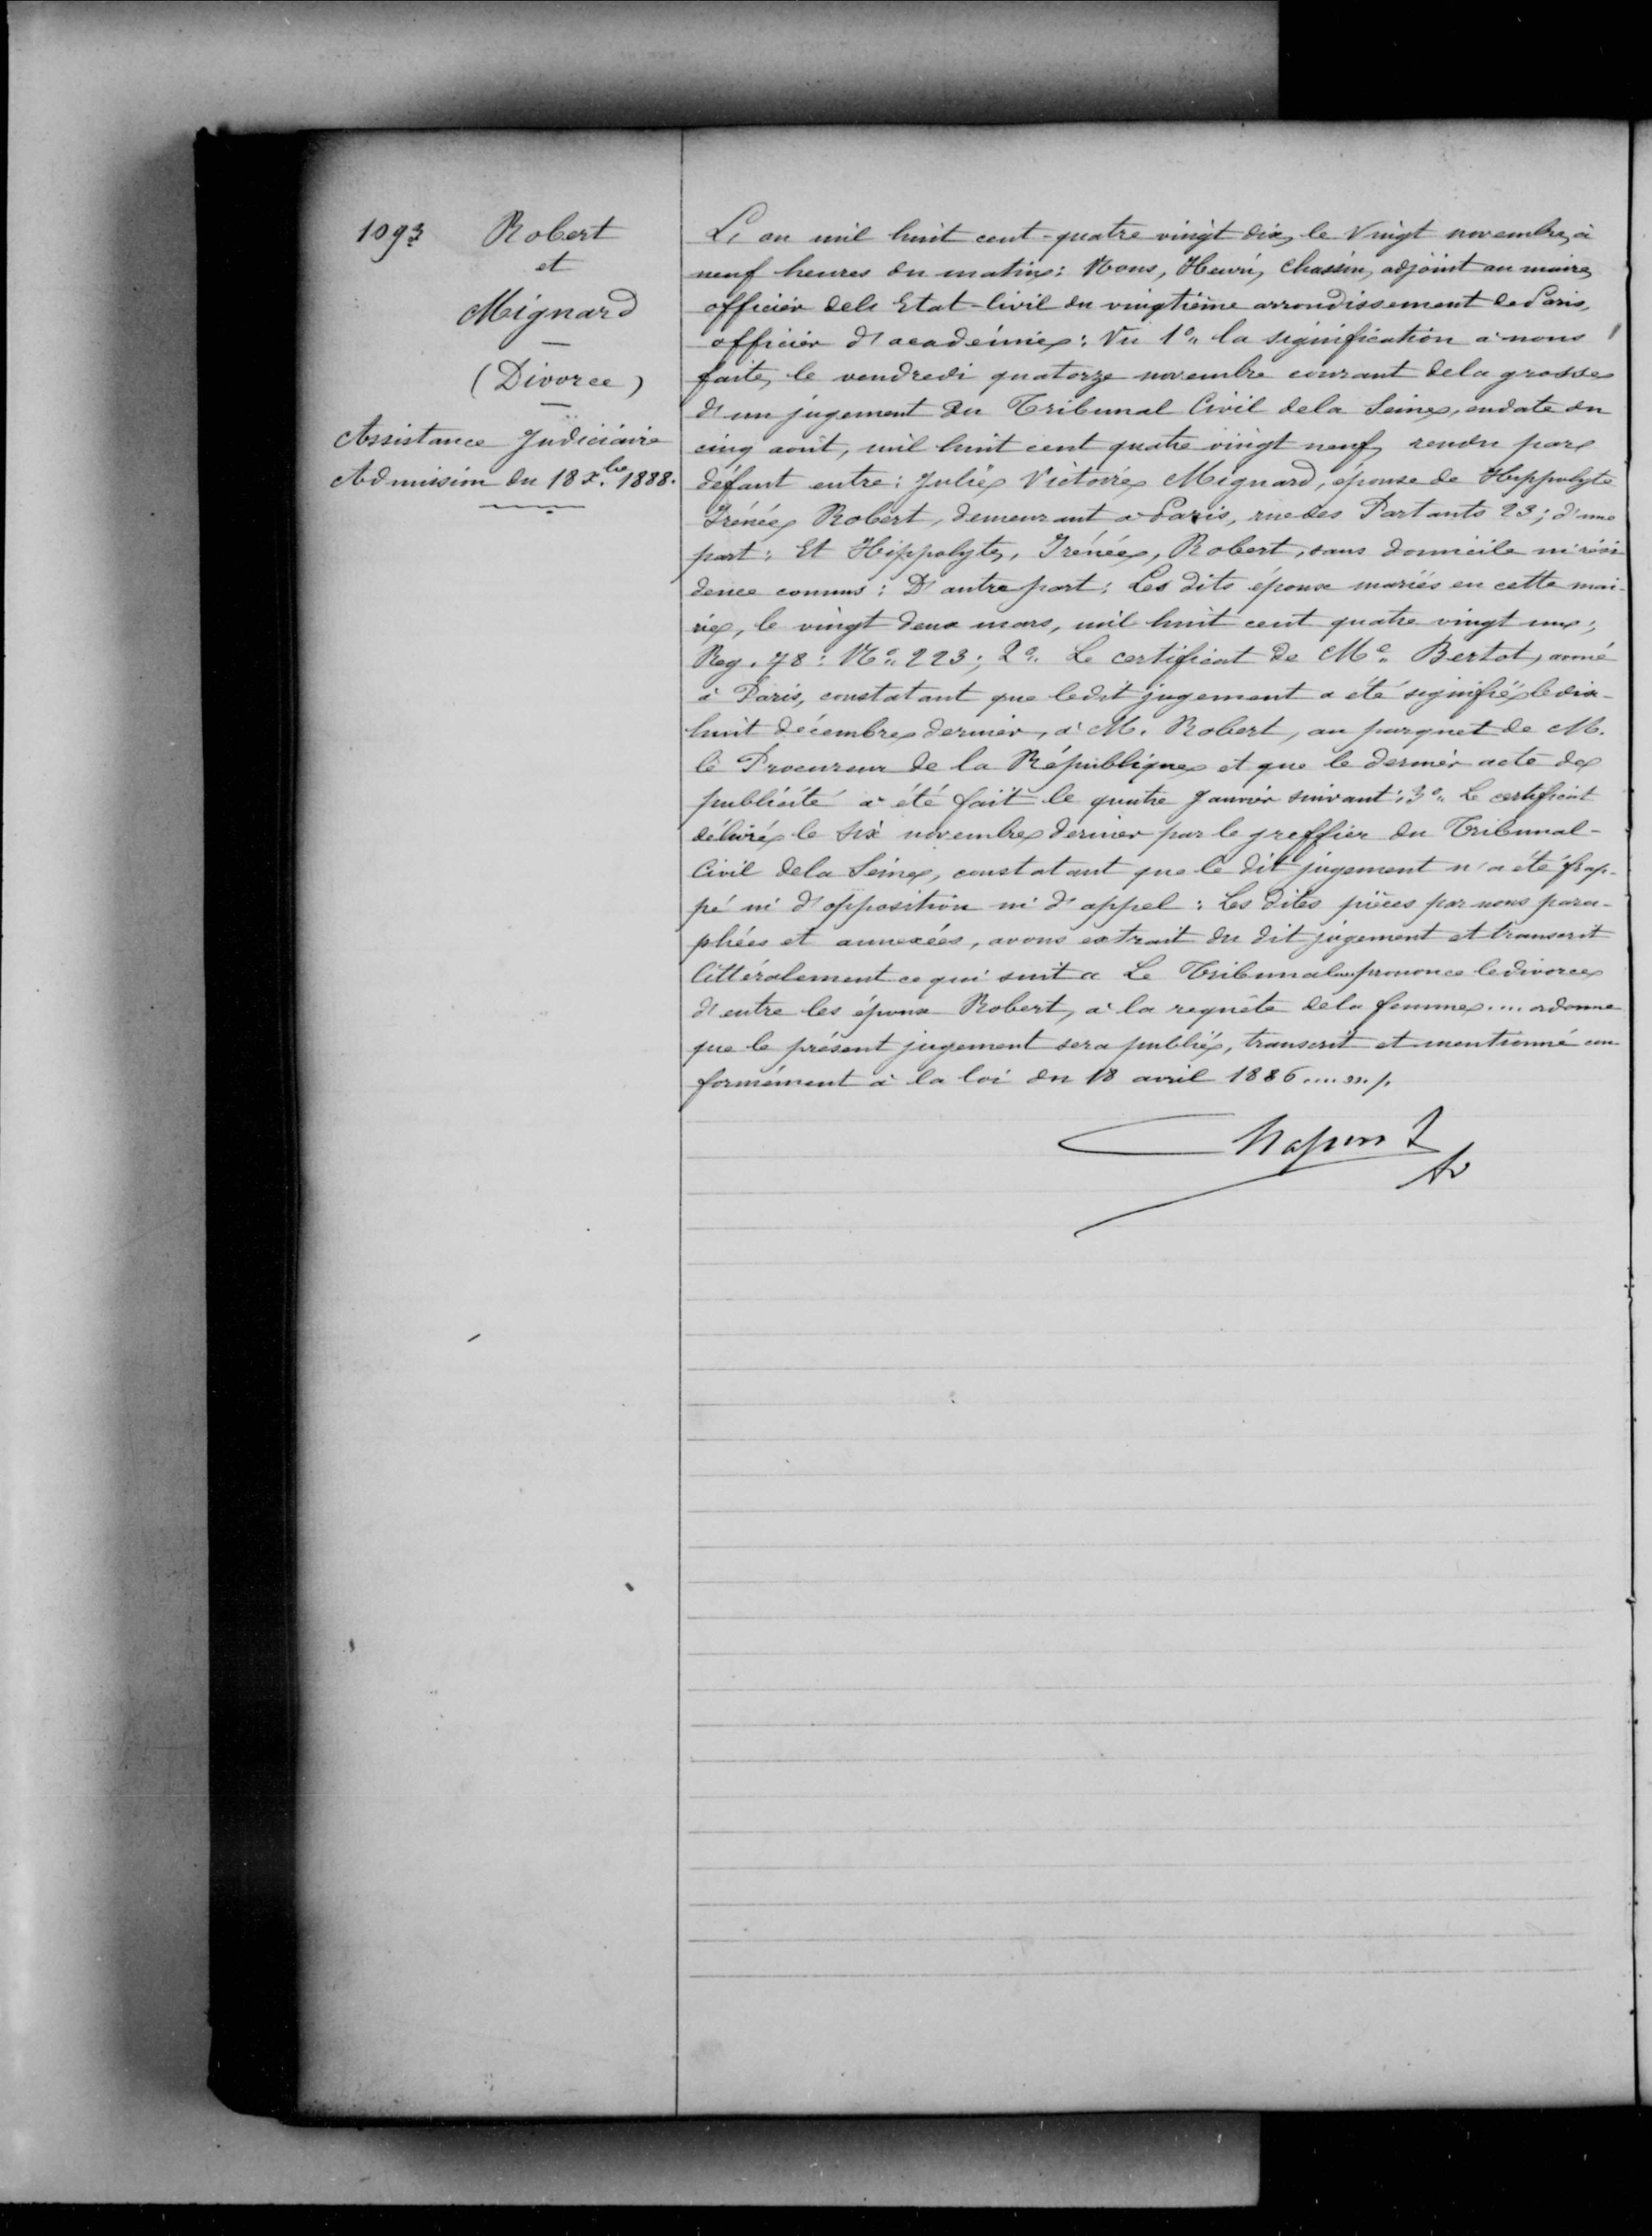1093 Robert
et
Mignard
-
(Divorce)
-
Assistance Judiciaire
Admision du 18x bis 1888
L'an mil huit cent quatre vingt dix, le Vingt novembre à
neuf heures du matin: Nous, Henri, Chassin, adjoint au maire
officier de l'Etat civil du vingtième arrondissement de Paris,
officier d'académie: Vu 1° la signification à nous
faite, le vendredi quatorze novembre courant de la grosse,
d'un jugement du Tribunal Civil de la Seine, en date du
cinq août, mil huit cent quatre vingt neuf, rendu par
défaut entre: Julie Victoire Mignard, épouse de Hippolyte
Irénée Robert, demeurant à Paris, rue des Portants 23; d'une
part: Et Hippolyte, Irénée, Robert, sans domicilie ni rési-
dence connus: D'autre part: Les dits époux mariés en cette mai-
rie, le vingt deux mars, mil uit cent quatre vingt deux;
Reg. 78: M°223; 29. Le certificat de M° Bertot, armé
à Paris, constatant que ledit jugement a ét signifié le dix-
huit décembre dernier, à M. Robert, au parquet de M.
le Procureur de la République et que le dernier acte de
publicité a été fait le quatre janvier suivant: 3°/ le certificat
délivré le dix novembre dernier par le greffier du Tribunal
Civil de la Seine, constatant que le dit jugement n'a été frap
pré ni d'opposition ni d'appel: Les dites pièces par nous para-
phées et annexées, avons extrait du dit jugement et transcrit
littéralement ce qui suit a Le Tribunal prononce le divorce
d'entre les époux Robert, à la requête de la femme .... ordonne
que le présent jugement sera publié, transcrit et mentionné con
formément à la loi du 18 avril 1886...../.
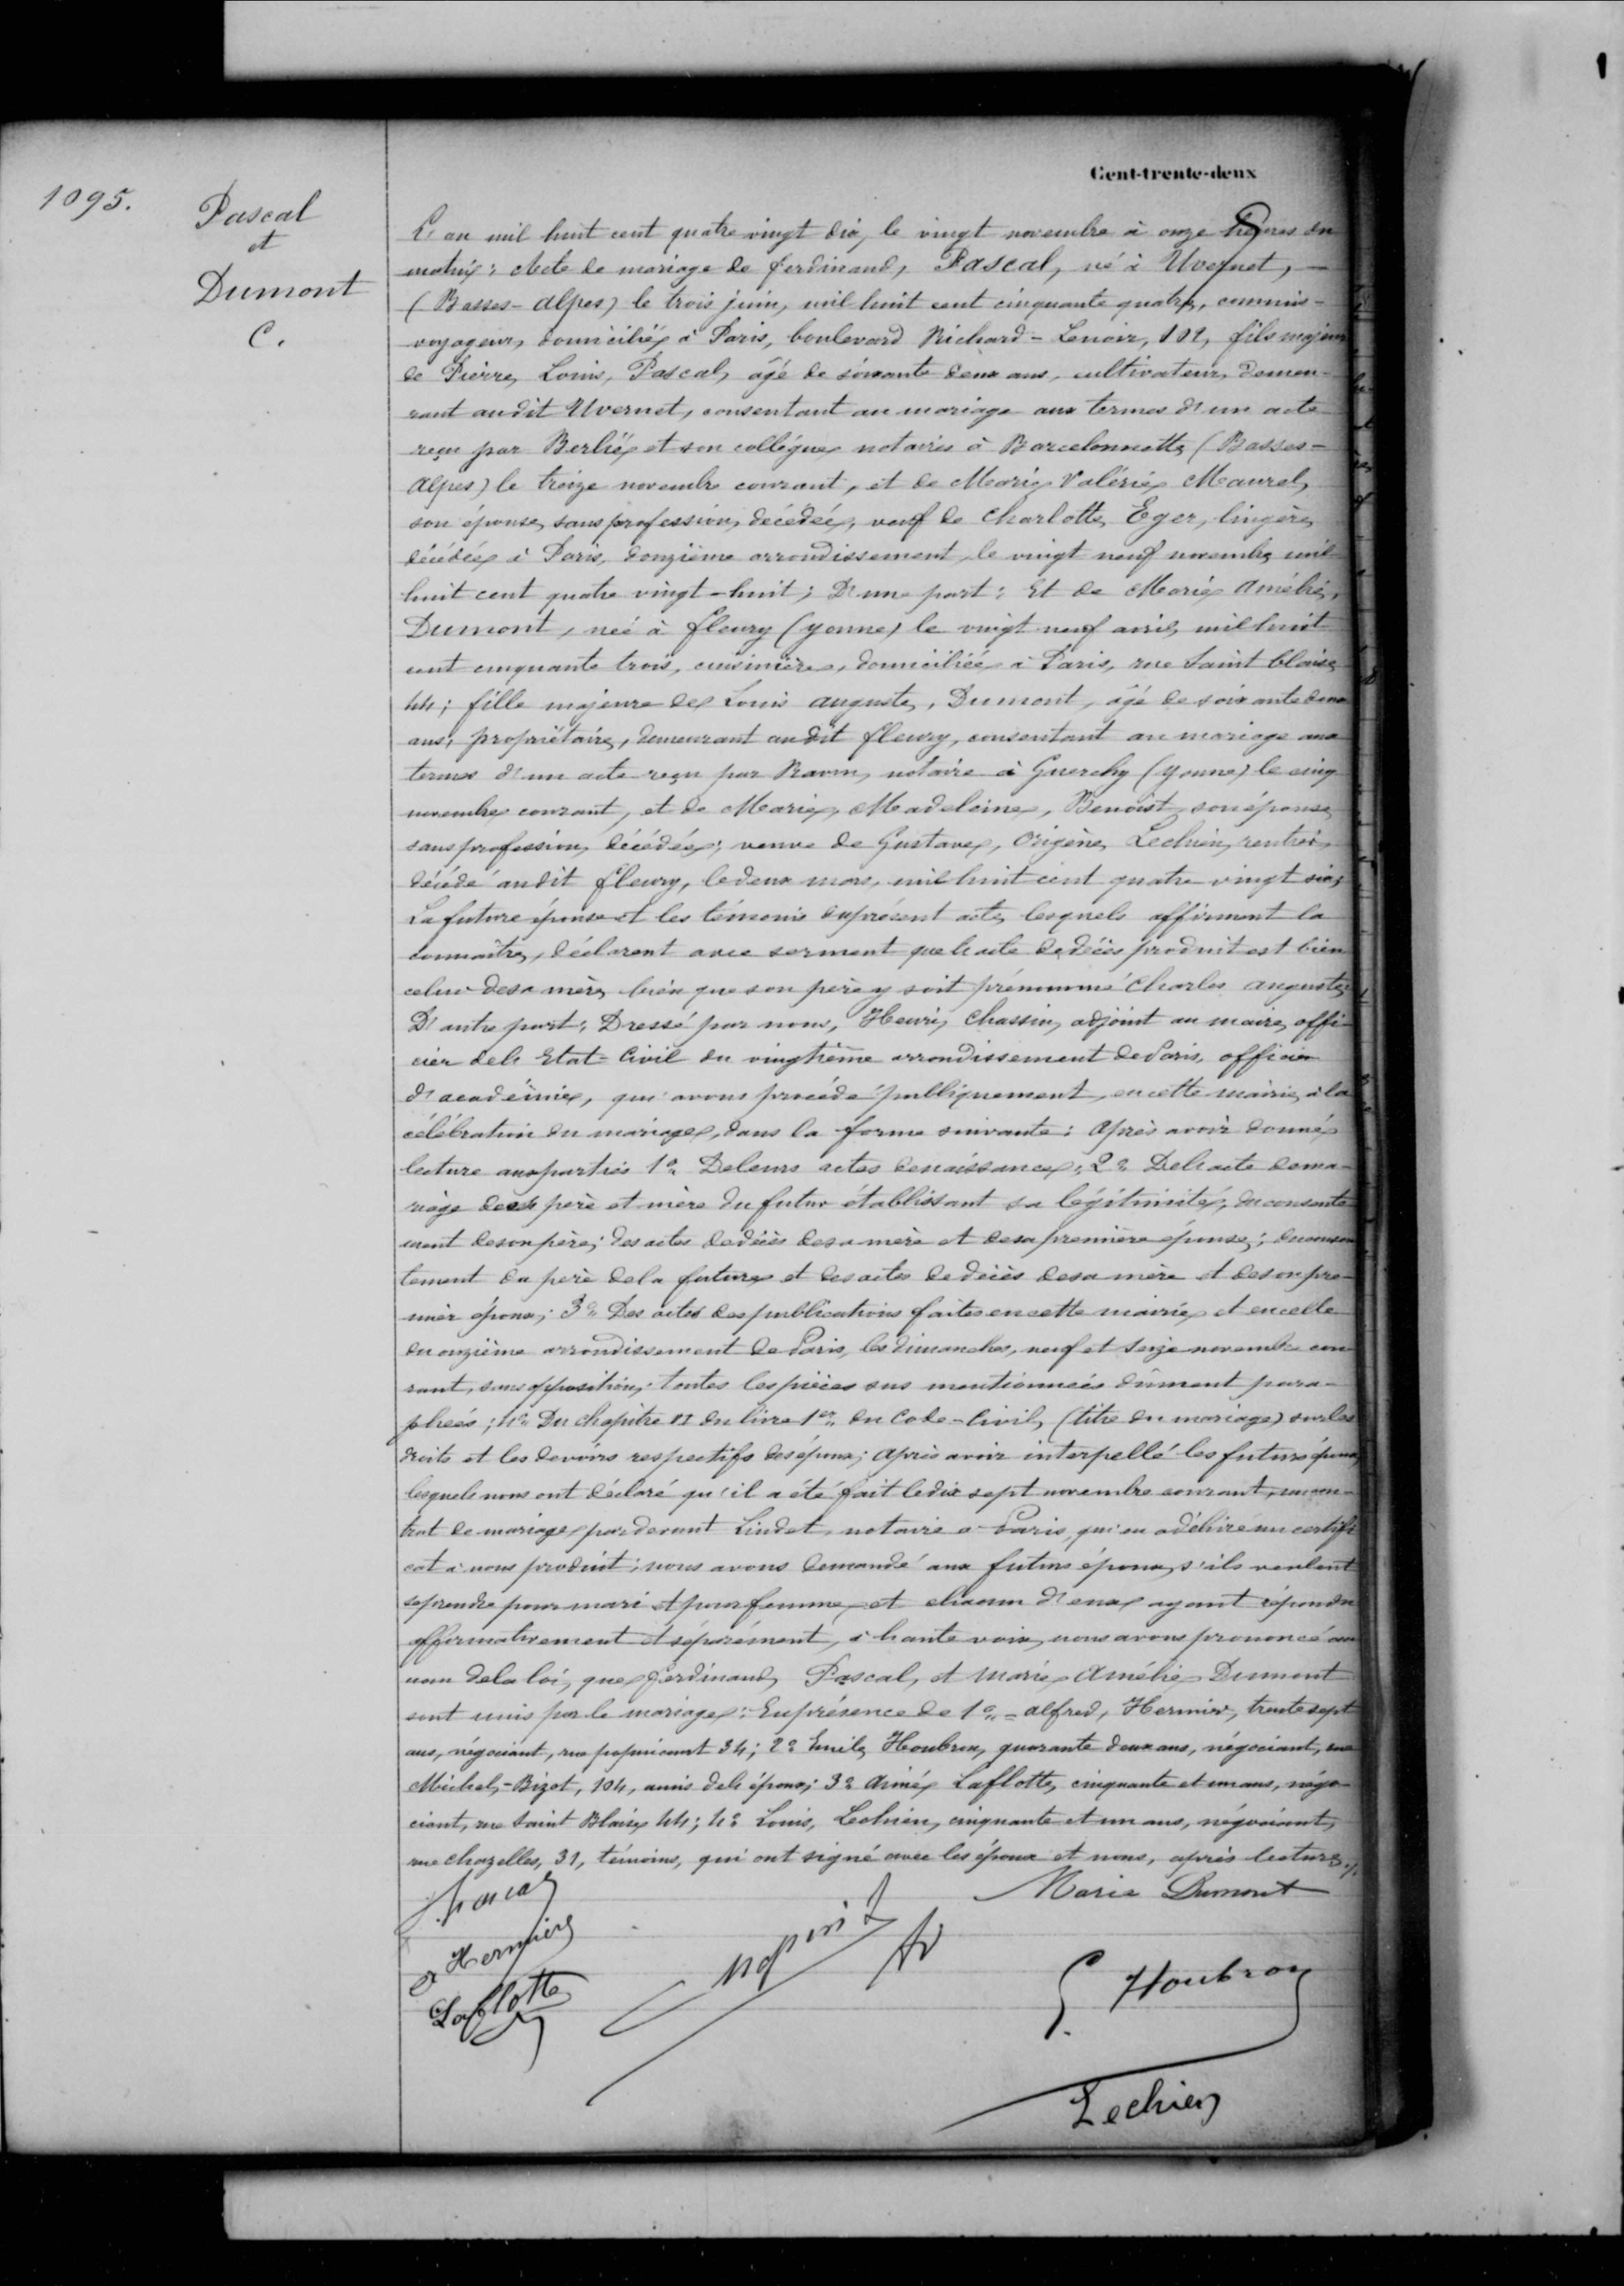1095
Pascal
et
Dumont
C.
L'an mil huit cent quatre vingt dix, le vingt novembre à onze heures du
matin: Acte de mariage de Ferdinand, Pascal, né à Uvernet, -
(Basses-Alpes) le trois juin, mil huit cent cinquante quatre, commis
voyageur, domicilié à Paris, boulevard Richard-Lenoir, 101, fils majeur
de Pierre, Louis, Pascal, âgé de soixante deux ans, cultivateur, demeu-
rant audit Uvernet, consentant au mariage aux termes d'un acte
reçu par Berlié et son collègue, notaire à Barcelonnette (Basses-
Alpes) le treize novembre courant, et de Marie Valérie Maurel,
son épouse, sans profession, décédée, veuf de Charlotte Eger, lingère
décédée à Paris, douzième arrondissement, le vingt neuf novembre mil
huit cent quatre vingt-huit; D'une part: Et de Marie Amélie,
Dumont, née à fleury (Yonne) le vingt neuf avril, mil huit
cent cinquante trois, cuisinière domiciliée à Paris, rue Saint Blaise
44; fille majeure de Louis auguste, Dumont, âgé de soixante deux
ans, propriétaire, demeurant audit fleury, consentant au mariage aux
termes d'un acte reçu par Ravin, notaire à Guerchy (Yonne) le cinq
novembre courant, et de Marie Madeleine, Benoist, son épouse
sans profession, décédés; veuve de Gustave, Origène, Leclin, rentier,
décédé audit fleury, le deux mars, mil hiut cent quatre vingt six;
La future épouse et les témoins du présent acte lesquels affirment la
connaitre, déclarent avec serment que l'acte de décès produit est bien
celui de sa mère bien que son père y soit prénommé Charles auguste
D'autre part: Dressé par nous, Henri, Chassin, adjoint au maire, offi
cier de l'Etat Civil du vingtième arrondissement de Paris, officier
d'académie, qui avons procédé publiquement en cette mairie à la
célébration du mariage dans la forme suivante: Après avoir donné
lecture aux parties 1°/ De leurs actes de naissance 2°/ de l'acte de ma-
riage des père et mère du futur établissant sa légitimité; des consente
ment de son père, des actes de décès des mère et de sa première épouse; du consen
tement du père de la future et les actes de décès de sa mère et de son pre-
mier époux; 3°: Des actes des publications faites en cette mairie et en celle
du onzième arrondissement de Paris, les dimanches, neuf et seize novembre cou
rants sans opposition: toutes les pièces sus mentionnées dûment para-
phées: 4°: Du chapitre VI du livre 1er du Code Civil (titre du mariage) sur les
droits et les devoirs respectifs des époux; Après avoir interpellé les futurs époux,
lesquels nous ont déclaré qu'il a été fait le dix septembre courant un con-
trat de mariage pardevant Lindet, notaire à Paris, qu'on a délivré un certifi
cat à nous produit; nous avons demandé aux futurs époux, s'ils veulent
se prendre pour mari et pour femme et chacun d'eux ayant répondu
affirmativement et séparément, à haute voix, nous avons prononcé au
nom de la loi, que Ferdinand, Pascal et Marie, Amélie, Dumont
sont unis par le mariage: En présence de 1° alfred, Hermise, trente sept
ans, négociant, rue popmicont 34; 2° Emile Houbron, quarante deux ans, négociant, rue
Michels Bizot, 104, amis de l'époux; 3° Aimé Lalfotte, cinquante et un ans négo-
ciant, rue Saint Blaise 44; 4° Louis, Lebien, cinquante et un ans, négociant,
rue chazelles, 31, témoins qui ont signé avec les époux et nous, après lecture ./.